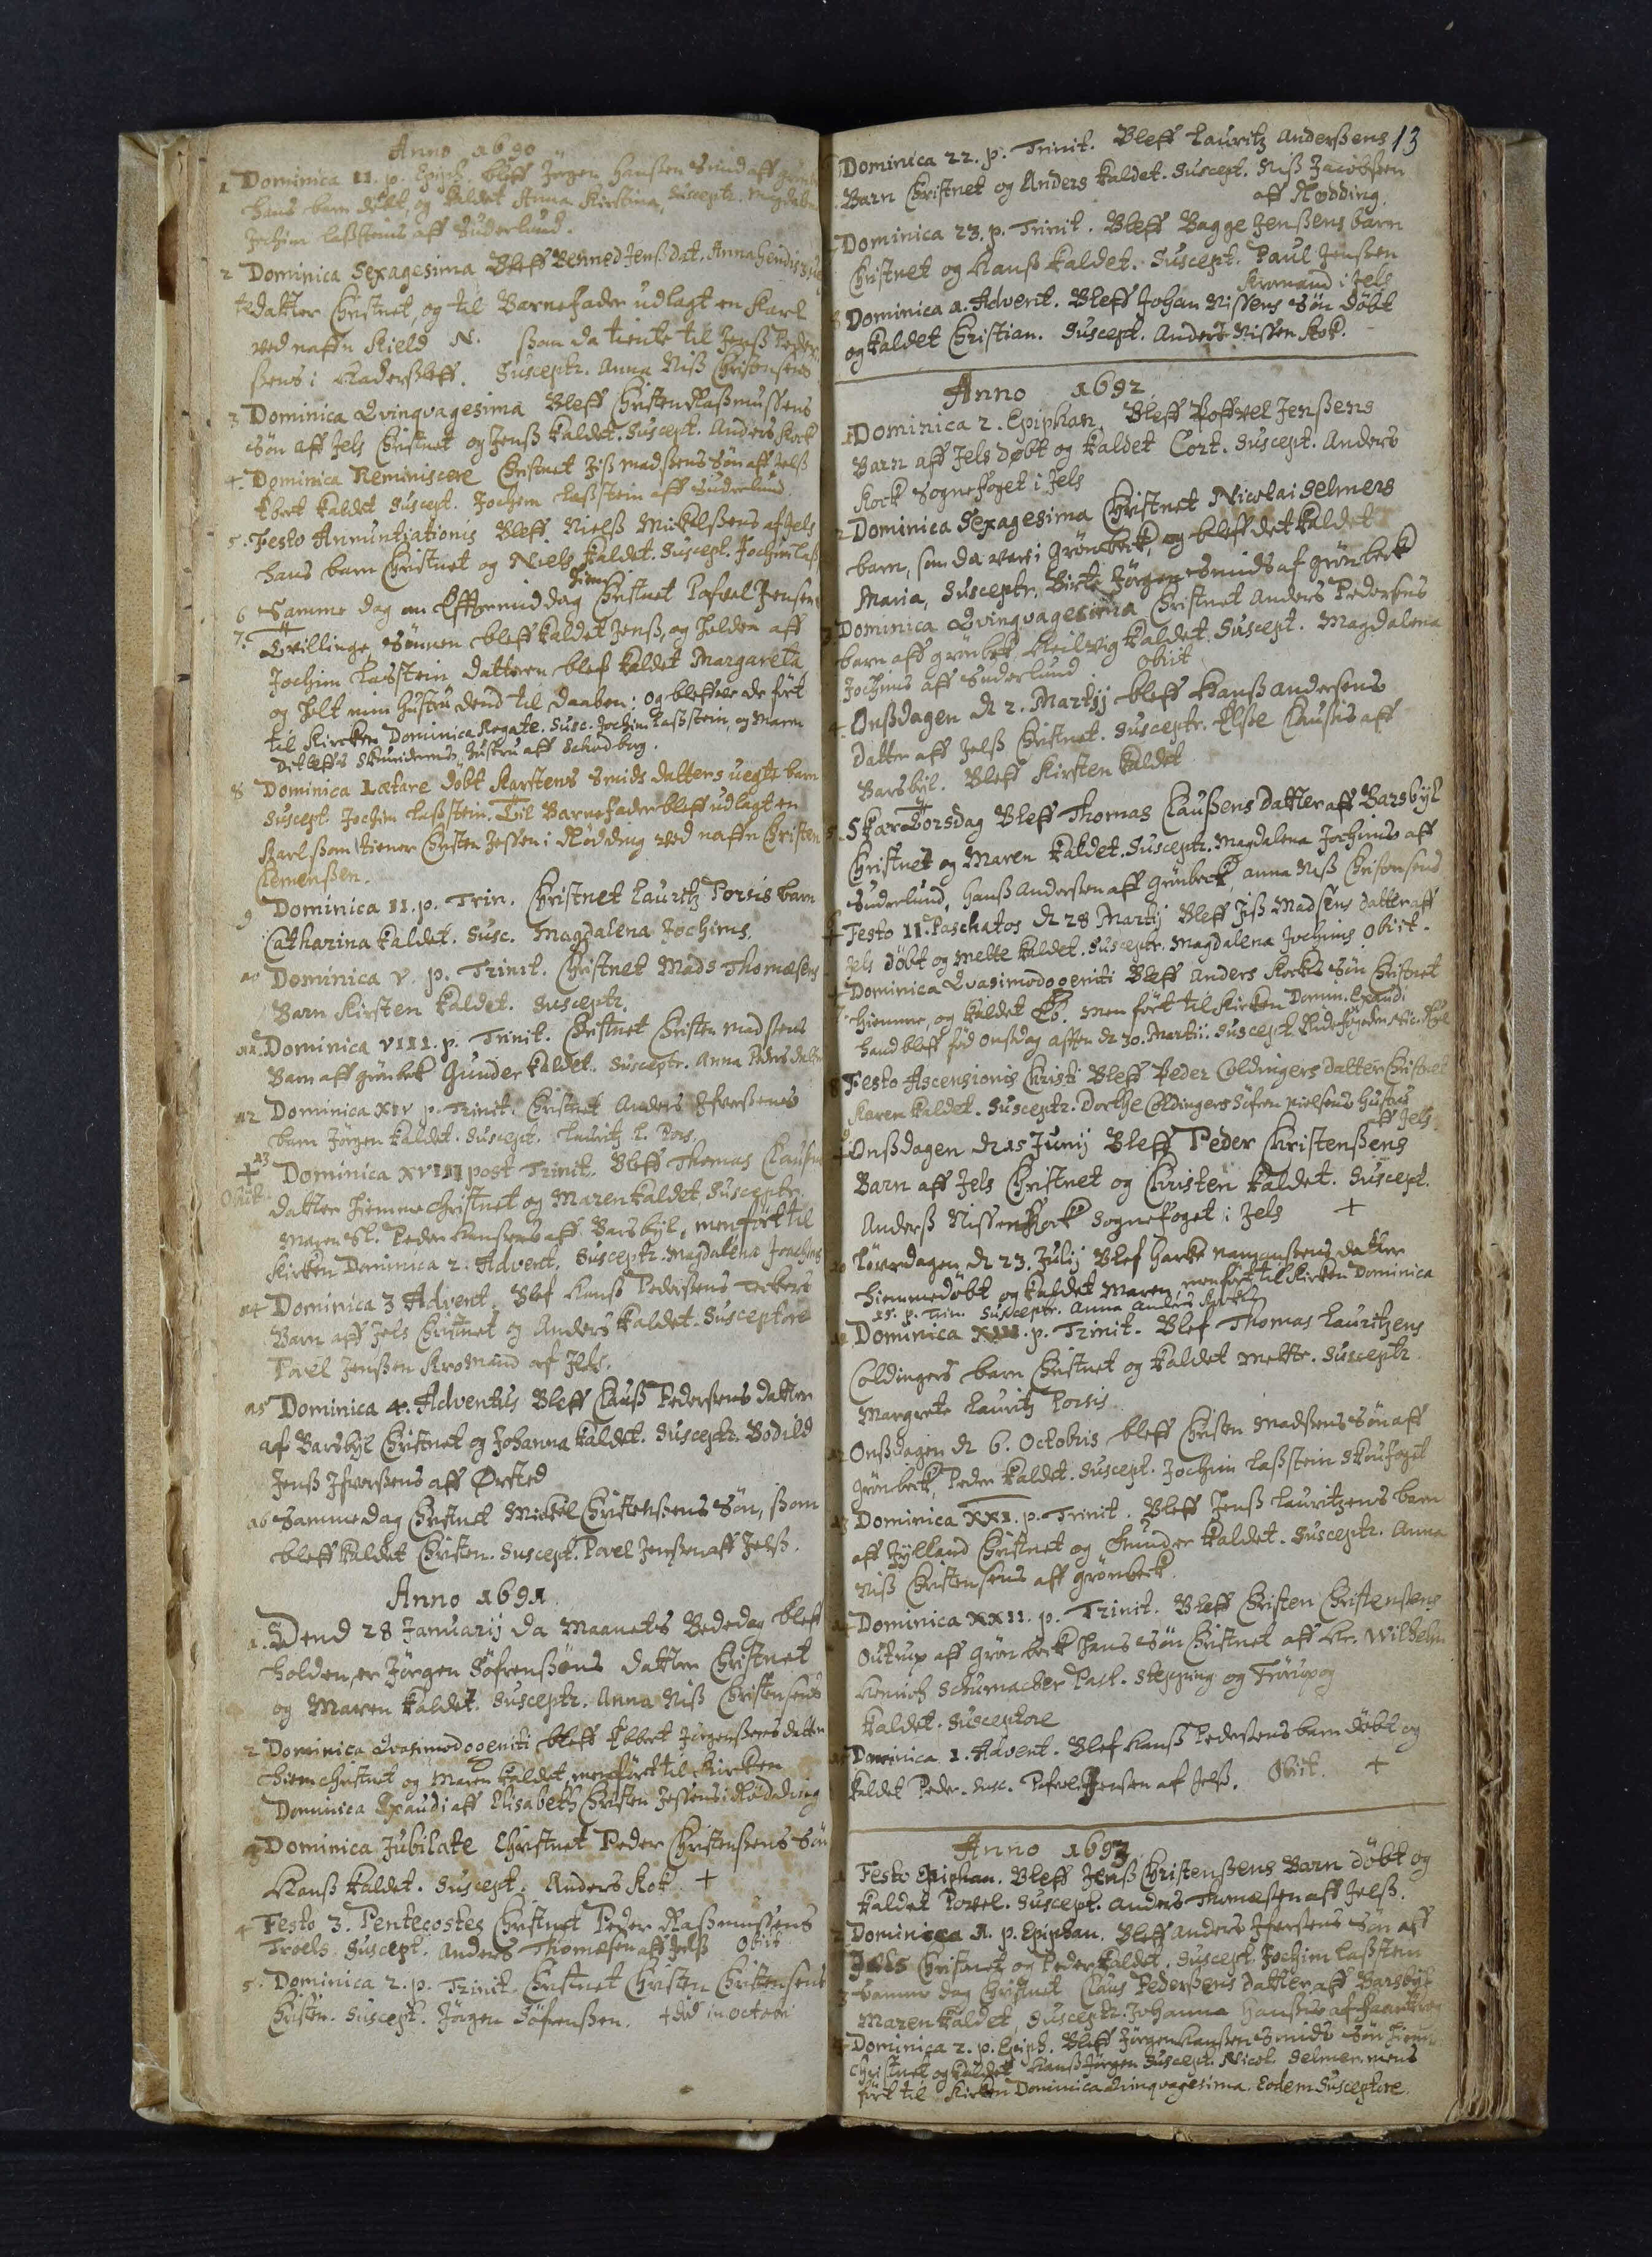Anno 1690
1 Dominica II p. Epiph. bleff Jørgen Hansen Smid aff Grønb
hans barn døbt, og kaldet Anna Kirstina, Suscept. Magdalene
Jochim Lassteins aff Suderlund.
2 Dominica Sexagesima Bleff Benned Jensssdat. Anna hendis ue
te Datter Christnet, og til Barnefader udlagt en Karl
ved naffn Kield N. som da tiente til Jenss Peder¬
sens i Hadersleff. Susceptr. Anna Niss Christensens
3 Dominica Qvinquagesima Bleff Christen Rasmussens
Søn aff Jels Christnet og Jenss kaldet. Suscept. Anders Kock
4. Dominica Reminoscere Christnet Jiss Madssens Søn aff Jelss
Ebert kaldet. Suscept. Jochem Lasstein aff Suderlund.
5. Festo Annuntiationis Bleff Niels Mickelsens af Jels
hans barn Christnet og Niels kaldet. Suscept. Jochim Lass 
Hiem##
6 Samme dag om eftermiddag Christnet Pofvel Jensens
7. Tvillinge Sønnen bleff kaldet Jens og holden aff
Jochim Lasstein Datteren bleff kaldet Margareta
og holt min Hustru dend til Daaben: og bleffve de ført
til Kircken Dominica Rogate . Susc. Jochim Lasstein og Maren
Detleffs Skouride## Hustru aff Schodborg
8 Dominica Lætare døbt Karstens Smids datters uegte barn
Suscept. Jochim Lasstein. Til Barnefader bleff udlagt en
karl som tiener Christen Jessen i Rødding ved naffn Christen
Clemensen.
9 Dominica II. p. Trin. Christnet Lauritz Porsis barn
Catharina kaldet. Susc. Magdalena Jochims.
10 Dominica V. p. Trinit. Christnet Mads Thomæsens
Barn Kirsten kaldet. Susceptr.
11. Dominica VIII. P. Trinit. Christnet Christen Madsens
Barn aff Grønbeck Gunder kaldet. Susceptr. Anna Pedersdatter
12 Dominica XIV p. Trinit. Christnet Anders Ifversens
barn Jørgen kaldet. Suscept. Lauritz L. Pors.
13
Dominica XVIII post Trinit. Bleff Thomas Clasens
datter hiemmechristnet og Maren kaldet. Susceptr. 
Maren Sl. Peder Hansens aff Barsbyl, men ført til
Kircken Dominica 2. Advent. Susceptr. Magdalena Jochims
14 Dominica 3 Advent. Bleff Hans Pedersens Teckers
Barn aff Jels Christnet og Anders kaldet. Susceptore
Povel Jensen Kromand af Jels.
15 Dominica 4. Adventus Bleff Claus Pedersens datter
af Barsbyl Christnet og Johanna kaldet. Susceptr. Bodild
Jens Ifversens aff Ørsted
16 Samme Dag Christnet Mickel Christensens Søn, som
bleff kaldet Christen. Suscept. Povel Jensen aff Jels.
Anno 1691
1. Dend 28 Januarij da Maaneds Bededag bleff
holden, er Jørgen Søfrenssens datter Christnet
og Maren kaldet. Susceptr. Anna Niss Christensens
2 Dominica Qvasimodogeniti bleff Ebbert Jørgenssens datter
hiemchristnet og Maren kaldet, men ført til Kirken
Dominica Exaudi aff Elisabeth Christen Jessens i Rødding
3 Dominica Jubilate Christnet Peder Christensens Søn
Hans kaldet. Suscept. Anders Kok.
4 Festo 3. Pentecostes Christnet Peder Rasmussens
Troels Suscept. Anders Thomæsen aff Jels. obiit
5. Dominica 2. p. Trinit. Christnet Christen Christensens
Christen. Suscept. Jørgen Søfrensen.
død in octobr
13
Dominica 22. p. Trinit. Bleff Lauritz Andersens
Barn Christnet og Anders kaldet. Suscept. Niss Jacobsen
aff Rødding.
Dominica 23 p. Trinit. Bleff Bagge Jensens barn
Christnet og Hans kaldet. Suscept. Paul Jensen
Kromand i Jels
8 Dominica I. Advent. Bleff Johan Nissens Søn døbt
og kaldet Christian. Suscept. Anders Nissen Kok.
Anno 1692
1 Dominica 2. Epiphan. Bleff Poffvel Jenssens
Barn aff Jels døbt og kaldet Cort. Suscept. Anders
Kock Sognefoget i Jels.
2 Dominica Sexagesima Christnet Nicolai Selmers
barn, som da var I Grønbeck, og bleff det kaldet
Maria, Susceptr. Birte Jørgen Smids af Grønbeck
Dominica Qvinquagesima Christnet Anders Pedersens
barn aff Grønbek Heilvig kaldet. Suscept. Magdalena
Jochims aff Suderlund. obiit
4. Onsdagen d 2. Martij bleff Hans Andersens
Datter aff Jels Christnet. Susceptr. Else Clausis aff
Barsbyl. Bleff Kirsten kaldet
5 Skar Torsdag Bleff Thomas Clausens datter aff Barsbyl
Christnet og Maren kaldet. Susceptr. Magdalena Jochims aff
Suderlund, Hans Andersen aff Grønbeck, Anna Niss Christensens
Festo II. Paschatos d 28 Martij Bleff Jiss Madsens datter aff
Jels døbt og Mette kaldet. Susceptr. Magdalena Jochims. obiit.
Dominica Qvasimodogeniti Bleff Anders Kockis Søn Christnet
7. hiemme, og kaldet Ib, men ført til Kirken Domin. Exaudi.
Hand bleff fød onsdag afften d 30. Martii. Suscept Ridefogeden Nic. Rye
Festo Ascensionis Christi Bleff Peder Coldingers datter Christnet
Karen kaldet. Susceptr. Dorthe Coldingers Søfren Nielsens hustru
aff Jels.
9.
Onsdagen d 15 Junij Bleff Peder Christensens
Barn aff Jels Christnet og Christen kaldet. Suscept.
Anders Nissen Kock sognefoget i Jels
10 Løverdagen d 23. Julij Blef Harke Namersens datter
hiemmedøbt og kaldet Maren, men ført til Kirken Dominica
15. p. Trin. Susceptr. Anna Anders Kockis
Dominica XIII p. Trinit. Blef Thomas Lauritzens
Coldingers barn Christnet og kaldet Mette. Susceptr
Margrete Lauritz Porsis
12. Onsdagen d 6. Octobris bleff Christen Madssens Søn aff
Grønbeck, Peder kaldet. Suscept. Jochim Lasstein Skoufoget
13 Dominica XXI. p. Trinit. Bleff Jenss Lauritzens barn
aff Jylland Christnet og Gunder kaldet. Susceptr. Anna
Niss Christensens aff Grønbeck.
14 Dominica XXII. p. Trinit. Bleff Christen Christensens
Outrup aff Grønbeck hans Søn Christnet aff Hr.. Wilhelm
Henrich Schumacher Past. Stepping og Frørup og
kaldet. Susceptore
15 Dominica I. Advent. Blef Hans Pedersens barn døbt og
kaldet Peder. Susc. Pofvel Jensen aff Jels. Obiit
Anno 1693
1 Festo Epiphan. Bleff Jens Christensens Barn døbt og
kaldet Povel. Suscept. Anders Thomæsen aff Jels.
2 Dominica 1. p. Epiphan. Bleff Anders Ifversens Søn aff
Jels Christnet og Peder kaldet. Suscept. Jochim Lasstein
3 Samme dag Christnet Claus Pedersens datter aff Barsbyl
Maren kaldet, Susceptr. Johanna Hansis af Faarkrog
4 Dominica 2. p. Epiph. Bleff Jürgen Hansen Smids Søn hiem
christnet og kaldet Hans Jørgen. Suscept. Nicol. Selmer, mens
ført til Kirken Dominica Qvinqvagesima. Eodem Susceptore.